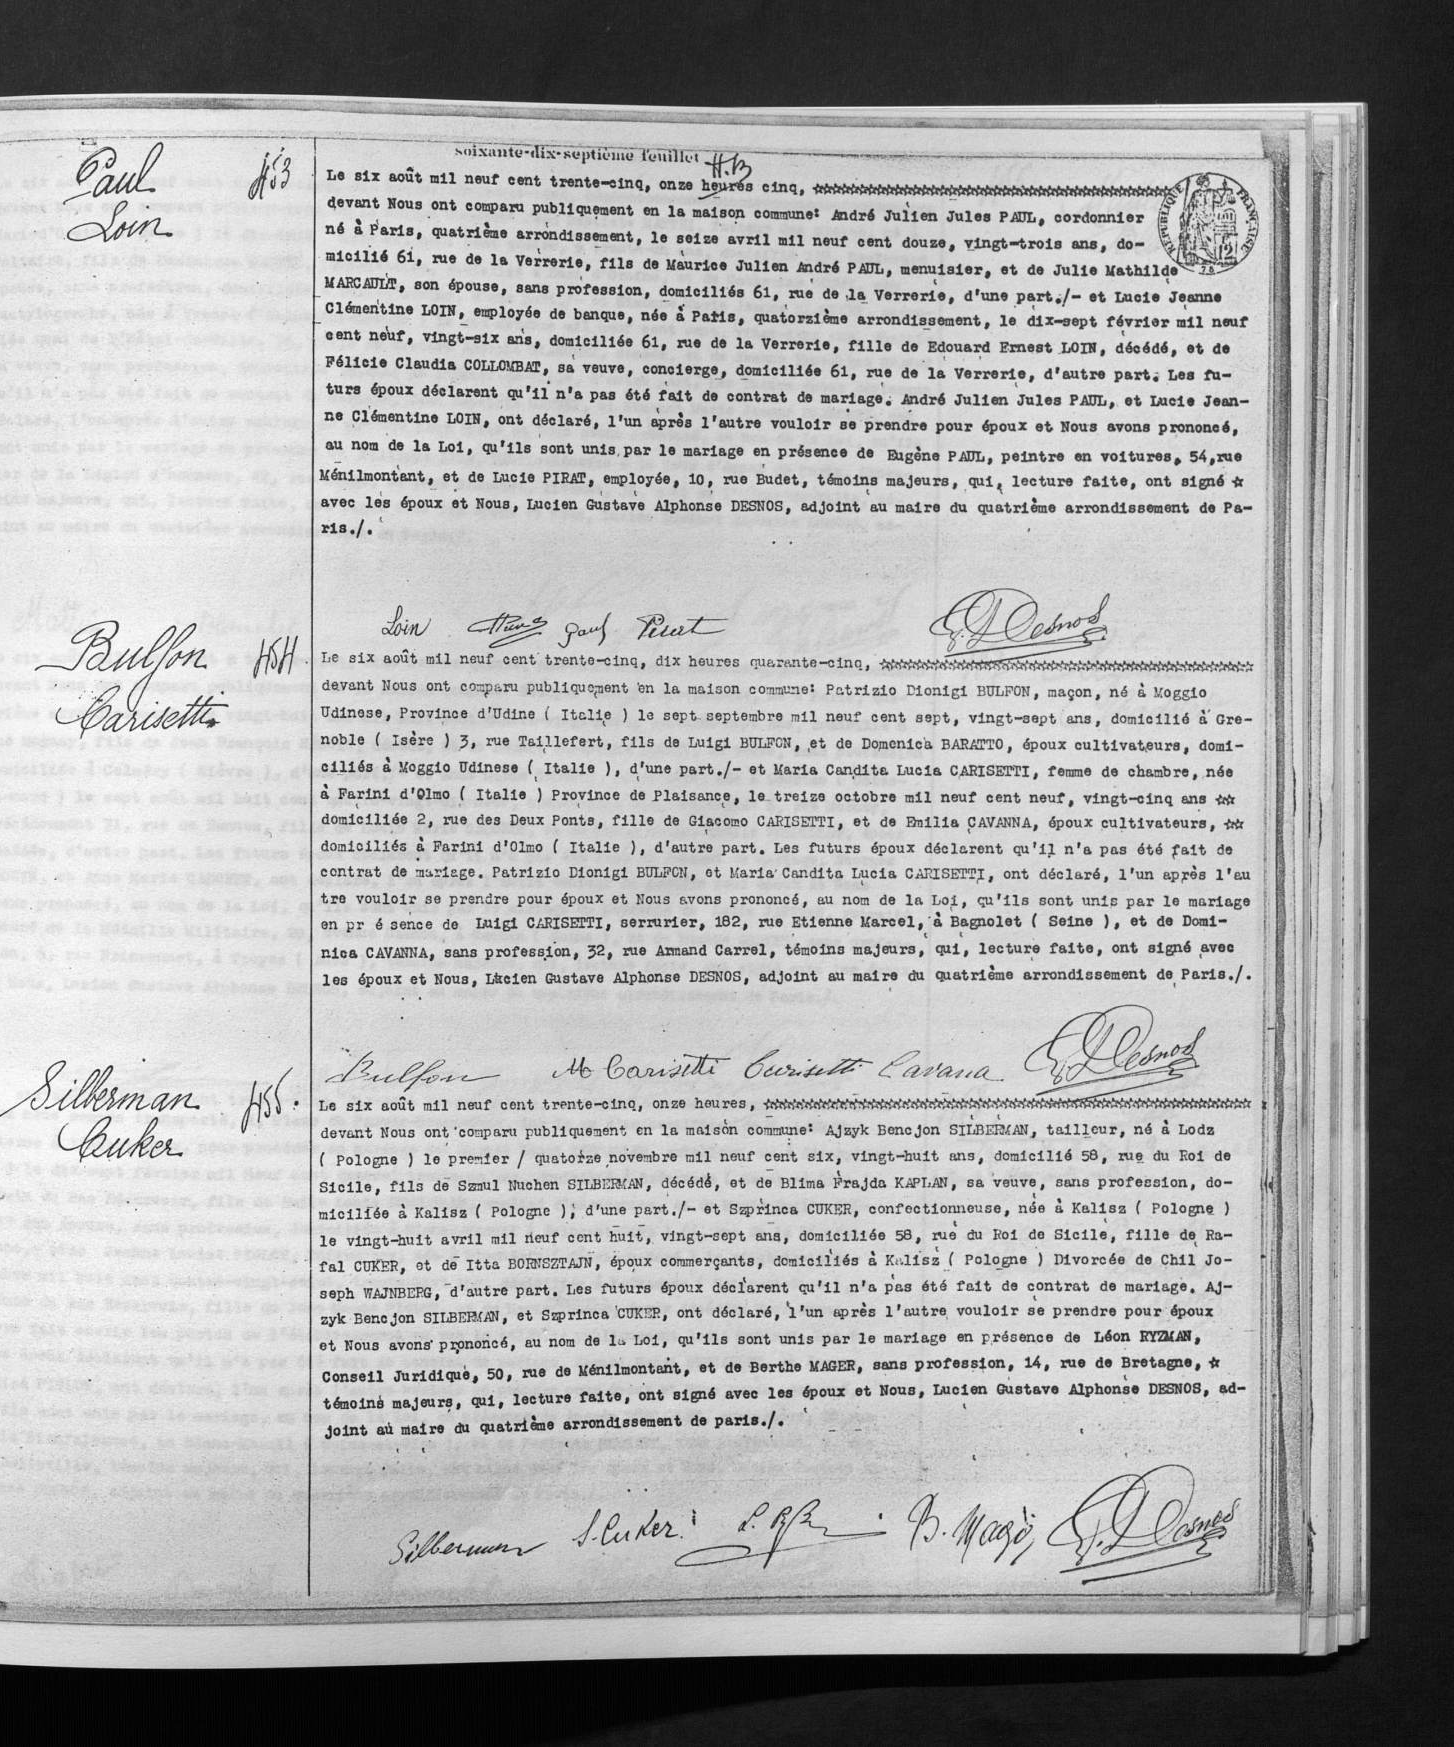Le six août mil neuf cent trente-cinq, onze heures cinq, ****
devant Nous ont comparu publiquement en la maison commune André Julien Jules PAUL, cordonnier
né à Paris, quatrième arrondissement, le seize avril mil neuf cent douze, vingt-trois ans, do-
micilié 61, rue de la Verrerie, fils de Maurice Julien André PAUL, menuisier, et de Julie Mathilde
MARCAULT, son épouse, sans profession, domiciliés 61, rue de la Verrerie, d'une part ./-Et Lucie Jeanne
Clémentine LOIN, employée de banque, née à Paris, quatorzième arrondissement, le dix-sept février mil neuf
cent neuf, vingt-six ans, domiciliée 61, rue de la Verrerie, fille de Edouard Ernest LOIN, décédé, et de
Félicie Claudia COLLOMBAT, sa veuve, concierge, domiciliée 61, rue de la Verrerie, d'autre part. Les fu-
turs époux déclarent qu'il n'a pas été fait de contrat de mariage. André Julien Jules PAUL, et Lucie Jean-
ne Clémentine LOIN, ont déclaré, l'un après l'autre vouloir se prenre pour époux et Nous avons prononcé,
au nom de la Loi qu'ils sont unis par le mariage en présence de Eugène PAUL, peintre en voitures, 54, rue
Ménilmontant, et de Lucie PIRAT, employée, 10, rue Budet, témoins majeurs, qui, lecture faite, ont signé *
avec les époux et Nous, Lucien Gustave Alphonse DESNOS, adjoint au maire du quatrième arrondissement de Pa-
ris ./.
Le six août mil neuf cent trente-cinq, dix heures quarante-cinq, ****
devant Nous ont comparu publiquement en la maison commune: Patrizio Dionigi BULFON, maçon, né à Moggio
Udinese, Province d'Udine (Italie) le sept septembre mil neuf cent sept, vingt-sept ans, domicilié à Gre-
noble (Isère) 3, rue Taillefert, fils de Luigi BULFON, et de Domenica BARATTO, époux cultivateurs, domi-
ciliés à Moggio Udines (Italie), d'une part ./-et Maria Candita Lucia CARISETTI, femme de chambre, née
à Farini d'Olmo (Italie) Province de Plaisance, le treize octobre mil neuf cent neuf, vingt-cinq ans **
domiciliée 2, rue des Deux Ponts, fille de Giacomo CARISETTI, et de Emilia CAVANNA, époux cultivateurs, **
domiciliés à Farini d'Olmo (Italie), d'autre part. Les futurs époux déclarent qu'il n'a pas été fait de
contrat de mariage. Patrizio Dionigi BULFON, et Maria Candita Lucia CARISETTI, ont déclaré, l'un après l'au
tre vouloir se prendre pour époux et Nous avons prononcé, au nom de la Loi, qu'ils sont unis par le mariage
en présence de Luigi CARISETTI, serrurie, 182, rue Etienne Marcel, à Bagnolet (Seine), et de Domi-
nica CAVANNA, sans profession, 32, rue Armand Carrel, témoins majeurs, qui, lecture faite, ont signé avec
les époux et Nous, Lucien Gustave Alphonse DESNOS, adjoint au maire du quatrième arrondissement de Paris ./.
Le six août mil neuf cent trente-cinq, onze heures, ****
devant Nous on comparu publiquement en la maison commune: Ajayk Bencjon SILBERMAN, tailleur, né à Lodz
(Pologne) le premier/ quatorze novembre mil neuf cent six, vingt-huit ans, domicilié 58, rue du Roi de
Sicile, fils de Szmul Nuchen SILBERMAN, décédé, et de Blima Frajda KAPLAN, sa veuve, sans profession, do-
miciliée à Kalisz (Pologne); d'une part ./-et Szprinca CUKER, conectionneuse, née à Kalisz (Pologne)
le vingt-huit avril mil neuf cent huit, vingt-sept ans, domiciliée 58, rue du Roi de Sicile, fille de Ra-
fal CUKER, et de Itta BORNSZTAJN, époux commerçants, domiciliés à Kalisz (Pologne) Divorcée de Chil Jo-
seph WAJNBERG, d'autre part. Les futurs époux déclarent qu'il n'a pas été fait de contrat de mariage. Aj-
zyk Bencjon SILBERMAN, et Szprinca CUKER, ont déclaré, l'un après l'autre vouloir se prendre pour époux
et Nous avons prononcé, au nom de la Loi, qu'ils sont unis par le mariage en présence de Léon RYZMAN,
Conseil Juridique, 50, rue de Ménilmontant, et de Berthe MAGER, sans profession, 14, rue de Bretagne, *
témoins majeurs, qui, lecture faite, ont signé avec les époux et Nous, Lucien Gustave Alphone DESNOS, ad-
joint au maire du quatrième arrondissement de paris ./.